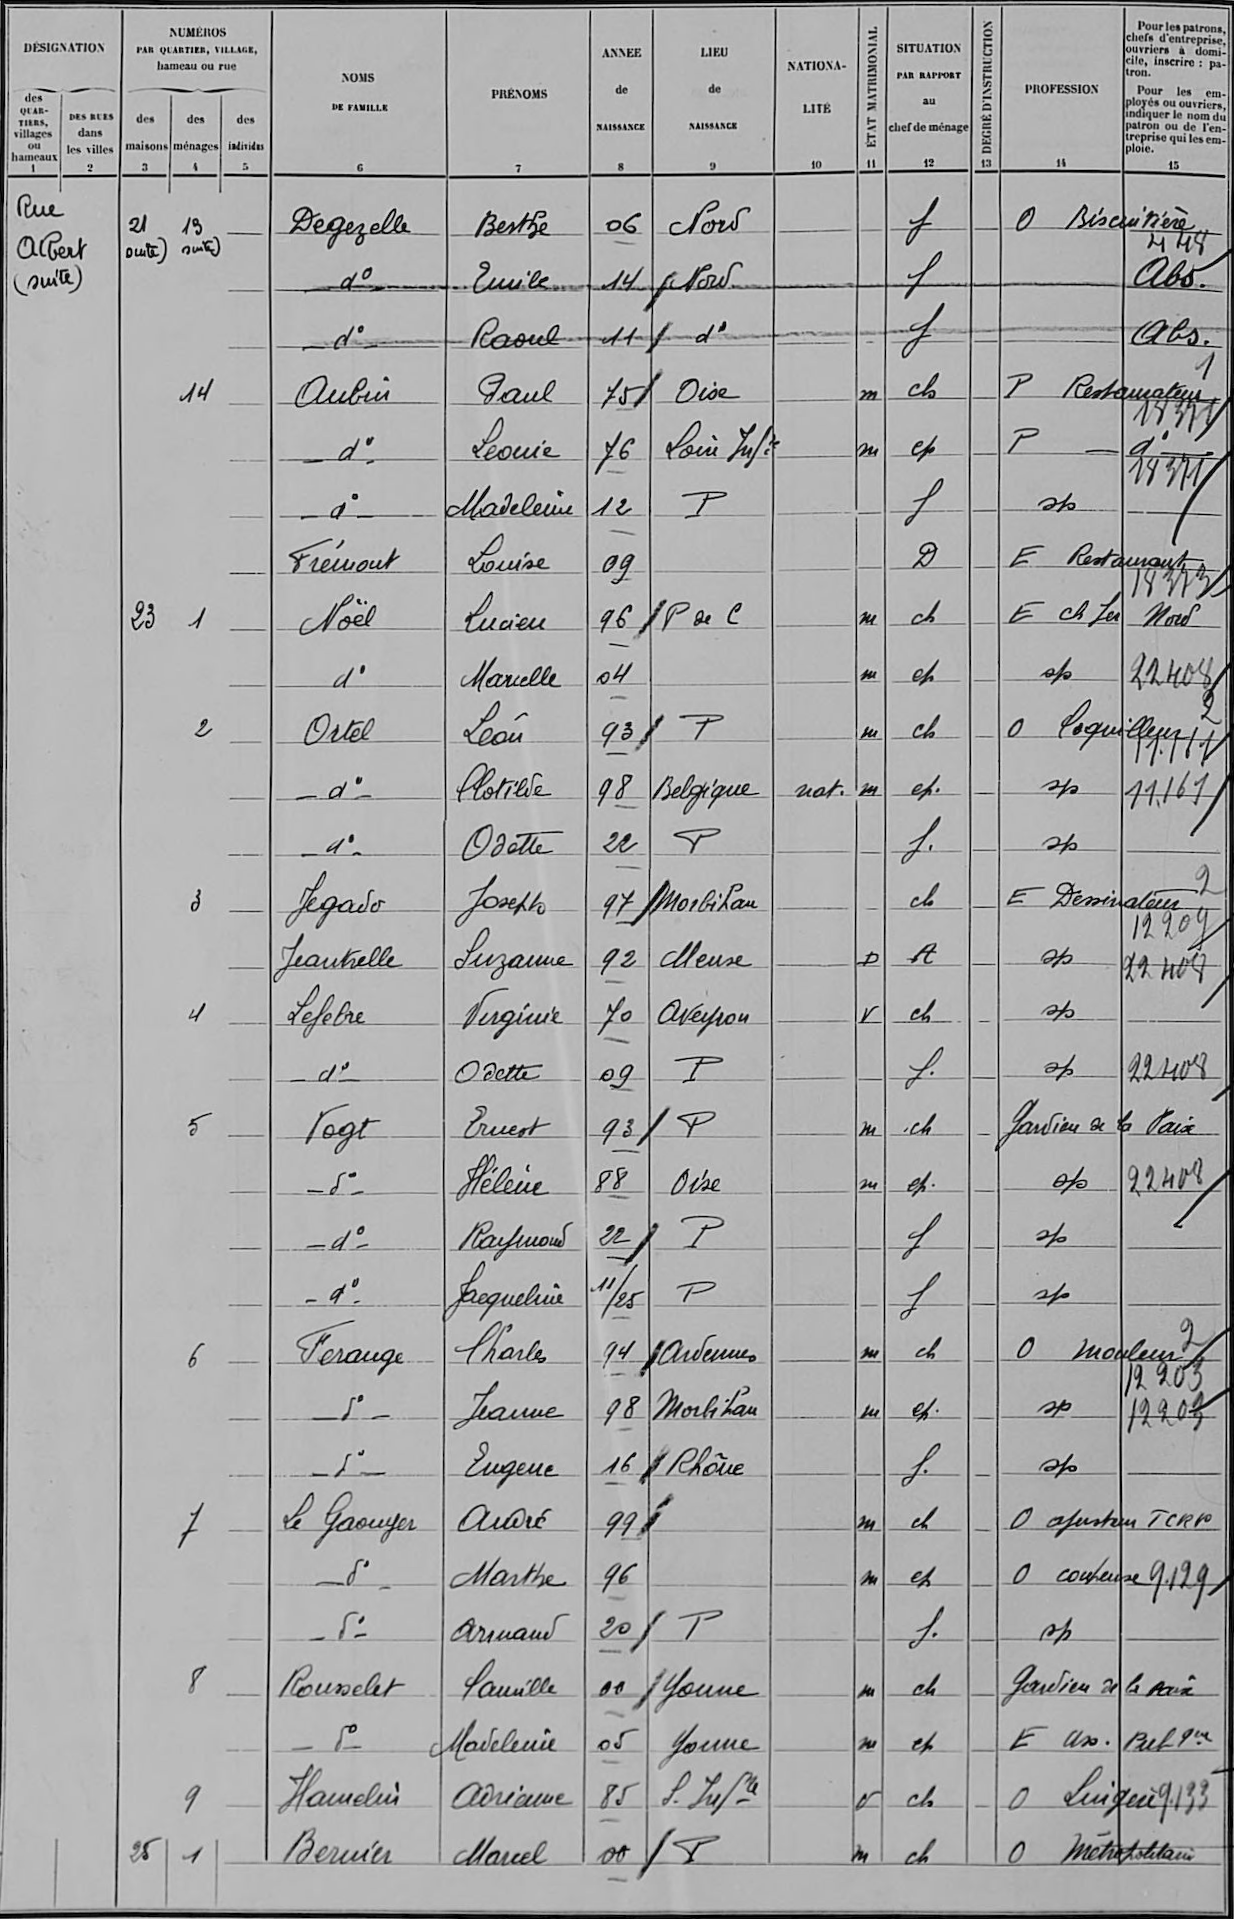Degezelle/Berthe/06/Nord/¤/¤/f/¤/O Biscuitière/448
d°/Emile/14/Nord/¤/¤/f/¤/¤/Abs
d°/Raoul/11/d°/¤/¤/f/¤/¤/Abs
Aubin/Paul/75/Oise/¤/m/ch/¤/P Restaurateur/!18371
d°/Leonie/76/Loire Infre/¤/m/ep/¤/P d°/!18371
d°/Madeleine/12/P/¤/¤/f/¤/sp/¤
Frémont/Louise/09/¤/¤/¤/D/¤/E Restaurant/!18373
Noël/Lucien/96/P de C/¤/m/ch/¤/E /ch fer Nord
d°/Marcelle/04/¤/¤/m/ep/¤/sp/22408
Ortel/Léon/93/P/¤/m/ch/¤/O Coquilleur/!11761
d°/Clotilde/98/Belgique/nat /m/ep /¤/sp/11161
d°/Odette/22/P/¤/¤/f /¤/sp/¤
Jegado/Joseph/97/Morbihan/¤/¤/ch/¤/E dessinateur/!12209
Jeantrelle/Suzanne/92/Meuse/¤/D/A/¤/sp/22408
Lefebre/Virginie/70/Aveyron/¤/V/ch/¤/sp/¤
d°/Odette/09/P/¤/¤/f /¤/sp/22408
Vogt/Ernest/93/P/¤/m/ch/¤/Gardien de la Paix/¤
d°/Hélène/88/Oise/¤/m/ep /¤/sp/22408
d°/Raymond/22/P/¤/¤/f/¤/sp/¤
d°/Jacqueline/25/P/¤/¤/f/¤/sp/¤
Ferange/Charles/94/Ardennes/¤/m/ch/¤/O mouleur/!12203
d°/Jeanne/98/Morbihan/¤/m/ep /¤/sp/12203
d°/Eugene/16/Rhône/¤/¤/f /¤/sp/¤
Le Gaouyer/André/99/¤/¤/m/ch/¤/O ajusteur/TCR V°
d°/Marthe/96/¤/¤/m/ep/¤/O couture/9129
d°/Armand/20/P/¤/¤/f /¤/sp/¤
Rousselet/Camille/00/Yonne/¤/m/ch/¤/Gardien de la Paix/¤
d°/Madeleine/05/Yonne/¤/m/ep/¤/E ass Pubque/¤
Hamelin/Adrienne/85/S Infre/¤/V/ch/¤/O Lingere/9133
Berurier/Marcel/00/P/¤/m/ch/¤/O métropolitain/¤
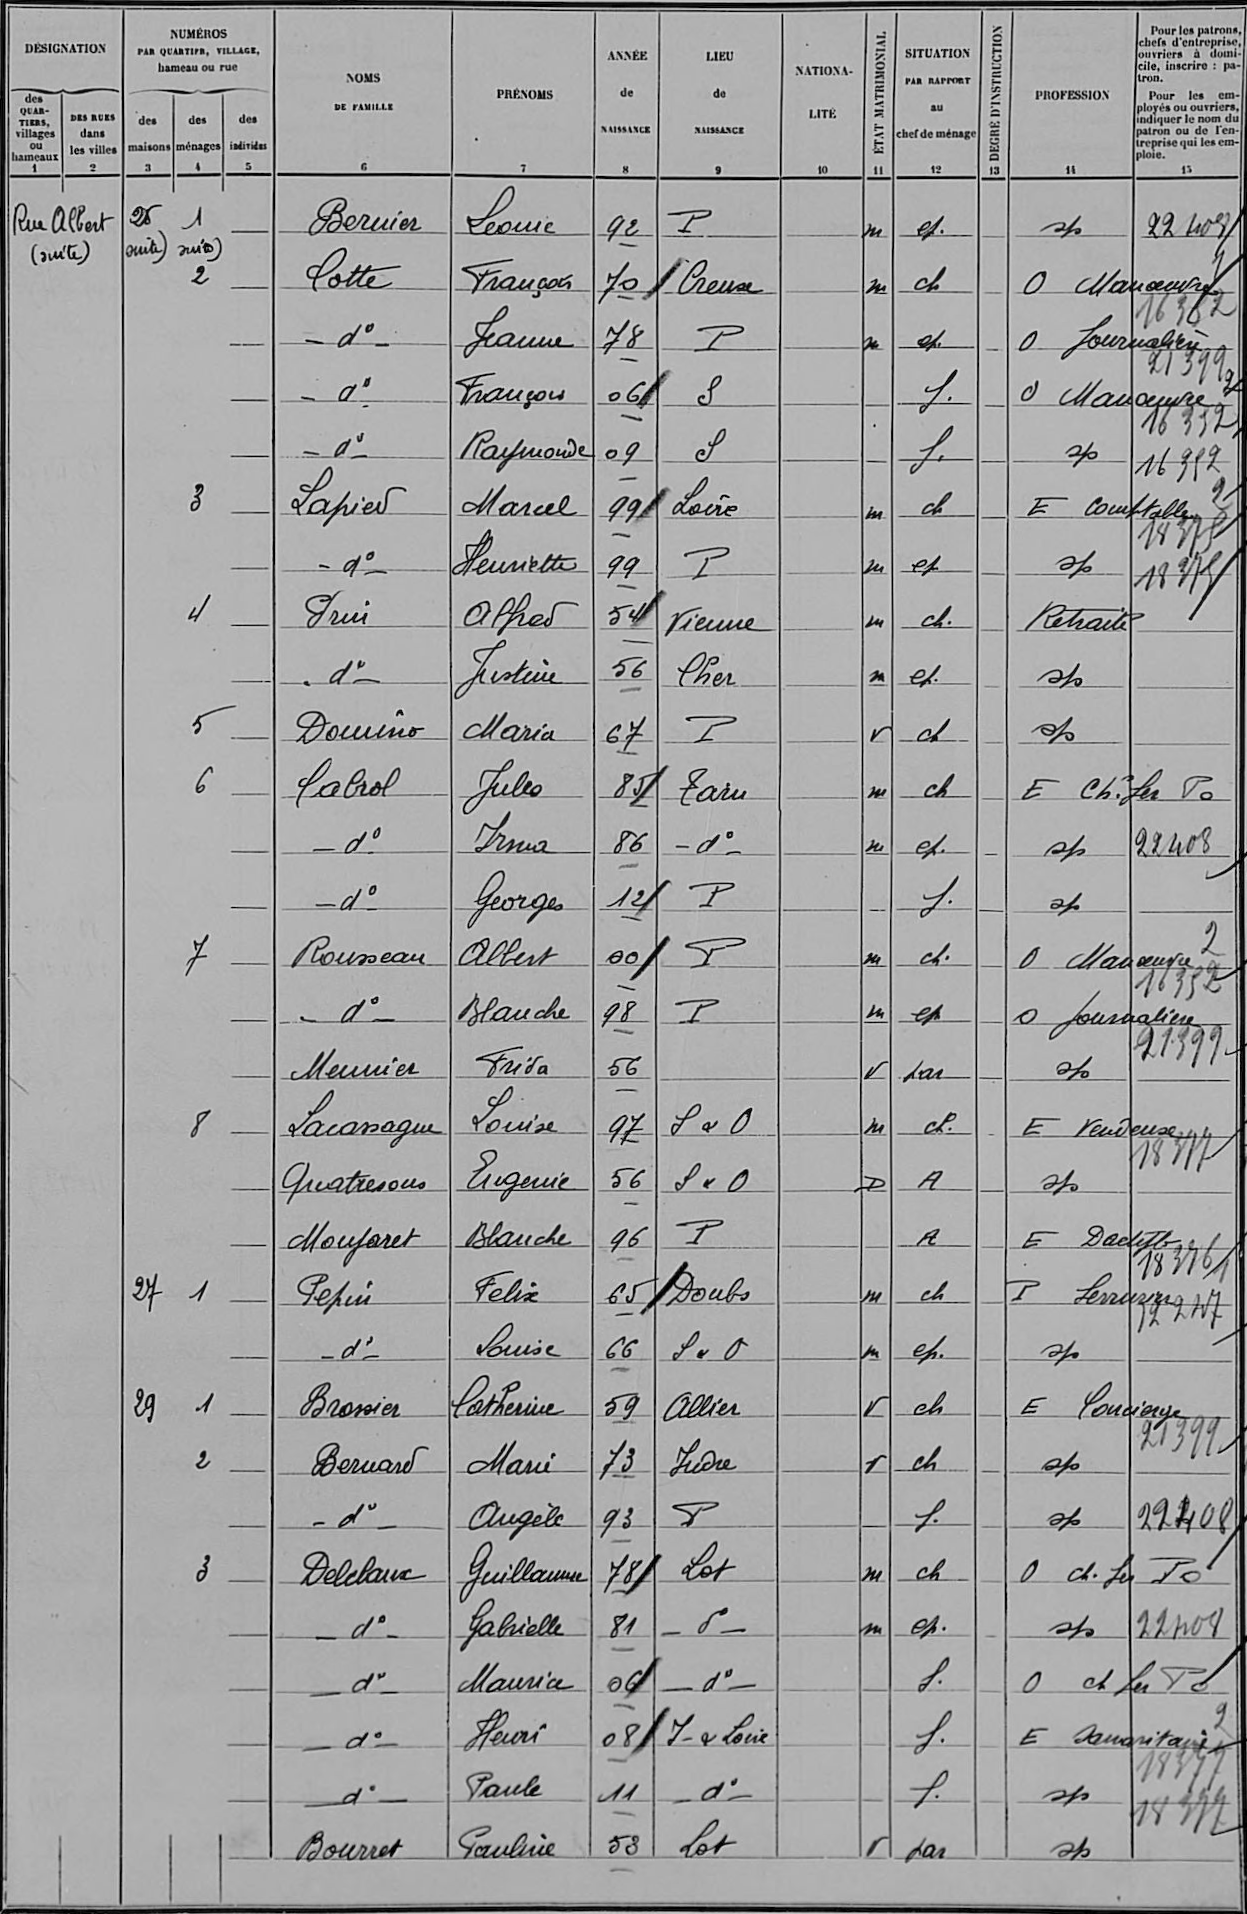Berurier/Leonie/92/P/¤/m/ep/¤/sp/22408
Cotte/François/70/Creuse/¤/m/ch/¤/O Manoeuvre/!16382
d°/Jeanne/78/P/¤/m/ep /¤/O Journaliere/!21399
d°/François/06/S/¤/¤/f /¤/O Manoeuvre/!16352
d°/Raymonde/09/S/¤/¤/f /¤/sp/16352
Lapiet/Marcel/99/Loire/¤/m/ch/¤/E comptable/!18375
d°/Henriette/99/P/¤/m/ep/¤/sp/18375
Prui/Alfred/54/Vienne/¤/m/ch /¤/Retraité/¤
d°/Justine/56/Cher/¤/m/ep/¤/sp/¤
Domino/Maria/67/P/¤/V/ch/¤/sp/¤
Cabrol/Jules/85/Tarn/¤/m/ch/¤/E chn fer/Po
d°/Irma/86/d°/¤/m/ep /¤/sp/22408
d°/Georges/12/P/¤/¤/f /¤/sp/¤
Rousseau/Albert/00/P/¤/m/ch /¤/O Manoeuvre/!16352
d°/Blanche/98/P/¤/m/ep/¤/O journaliere/!21399
Meunier/Frida/56/¤/¤/V/par/¤/sp/21399
Lacassagne/Louise/97/S & O/¤/m/ch /¤/E vendeuse/!18377
Quatresous/Eugenie/56/S & O/¤/D/A/¤/sp/¤
Moujaret/Blanche/96/P/¤/¤/A/¤/E dactylo/!18376
Pepin/Felix/65/Doubs/¤/m/ch/¤/P Serrurier/!12 227
d°/Louise/66/S & O/¤/m/ep /¤/sp/¤
Brossier/Catherine/59/Allier/¤/V/ch/¤/E Concierge/!21399
Bernard/Marie/73/Indre/¤/V/ch/¤/sp/¤
d°/Angèle/93/P/¤/¤/f /¤/sp/22408
Delelaux/Guillaume/78/Lot/¤/m/ch/¤/O ch fer/Po
d°/Gabrielle/81/d°/¤/m/ep /¤/sp/22408
d°/Maurice/06/d°/¤/¤/f /¤/O ch fer/Po
d°/Henri/08/I & Loire/¤/¤/f /¤/E samaritaine/!18399
d°/Paule/11/d°/¤/¤/f /¤/sp/18377
Bourret/Pauline/53/Lot/¤/V/par/¤/sp/¤